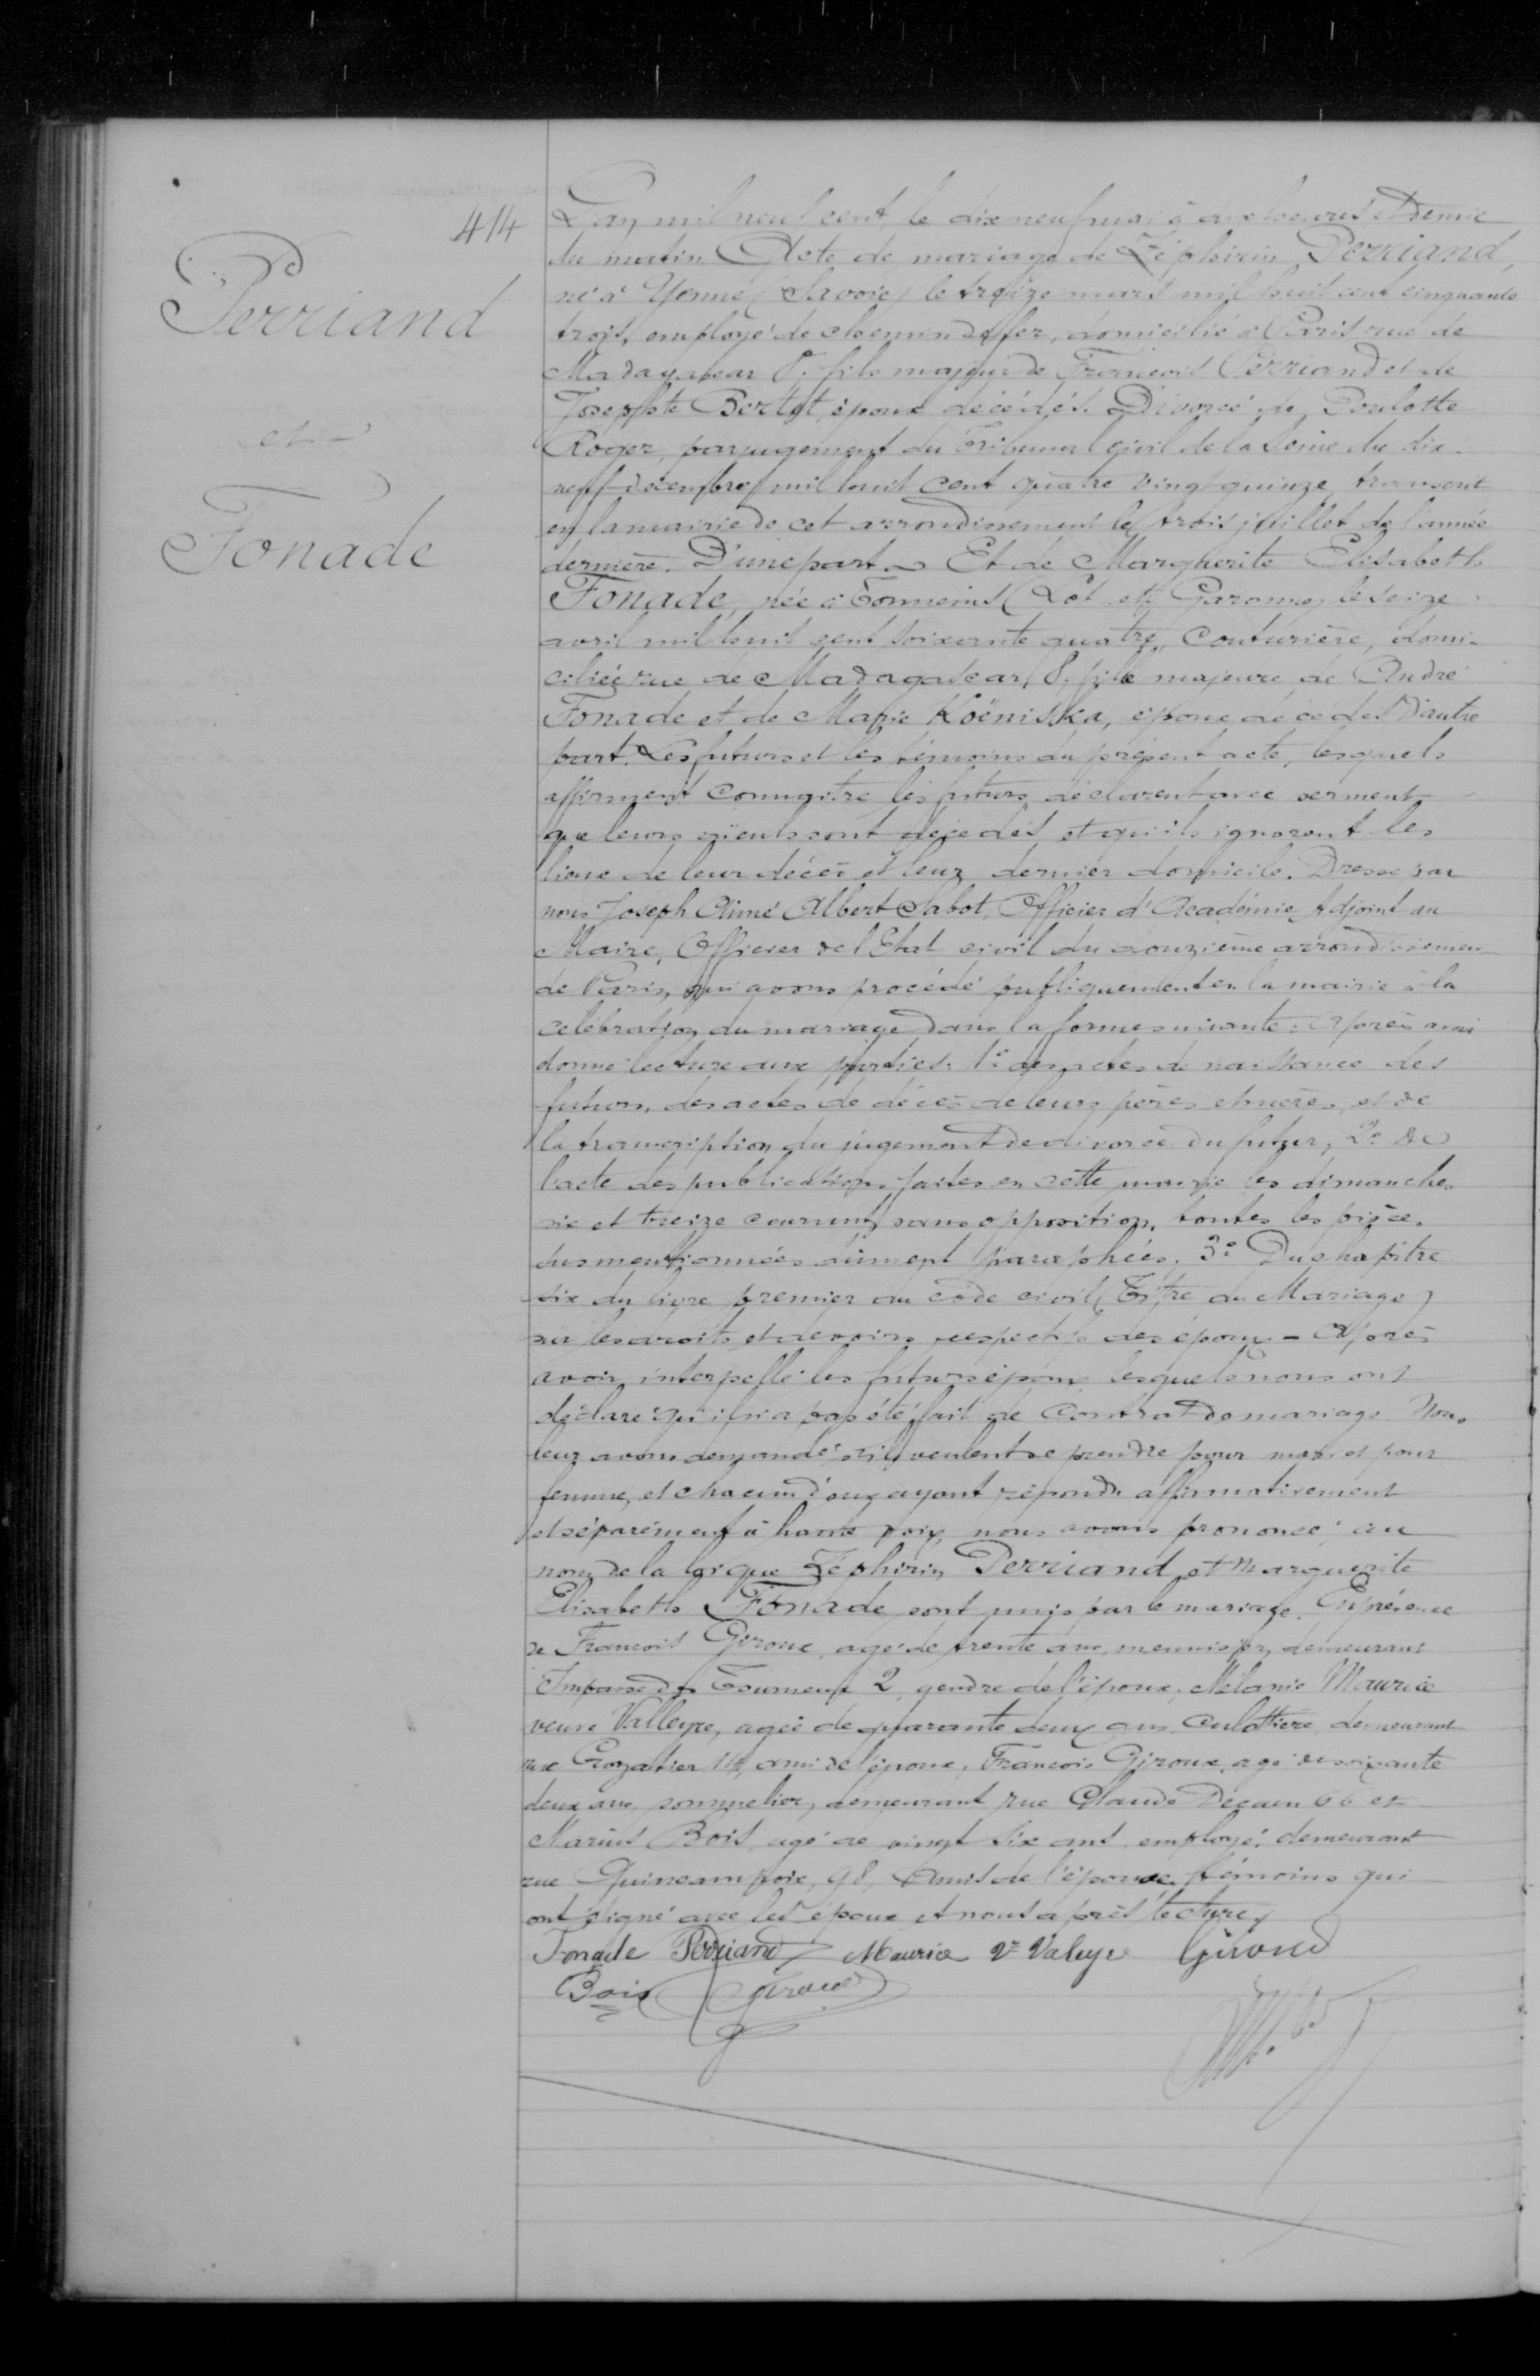414
Perriand
et
Fonade
L'an mil neuf cent le dix neuf mai à dix heures et Demie
du matin Acte de mariage de Zéphirin Perriand,
né à Yonne (Saone) le treize mars mil huit cent cinquante
trois, employé de chemin de fer, domicilié à Paris rue de
Madagascar fils majeur de François Perriand et de
Josephte Bertot, époux décédés. Divorcé de Porlotte
Roger, par jugement du Tribunal civil de la Seine du dix
neuf decembre mil huit cent quatre vingt quinze transcrit
en la mairie de cet arrondissement le trois juillet de l'année
dernière. D'une part. Et de Marguerite Elisabeth
Fonade, née à Tonnems (Lôt et Garonne) le seize
avril mil huit cent soixant quatre couturière domi-
ciliée rue de Madagascar 8, fille majeure de André
Fonade et de Marie Koeniska, epoux décédé et d'autre
part Les futurs et les témoins du présent acte, lesquels
affirmant connaitre les futurs déclarent avec serments
que leurs aïeuls sont décédé et qu'ils ignorent les
lieux de leur décès et leur dernier domicilie. Dressé par
nous Joseph Aimé Albert Labot, Officier d'Académie Adjoint au
Maire, Officier de l'Etat-Civil du douzième arrondissement
de Paris, qui avons procédé publiquement en la mairie à la
célébration du mariage dans la forme suivante: Après avoir
donne lecture aux parties 1° des actes de naissance des
fonctions, des actes de décès de leurs pères et mères et de
la transcription du jugement de divorce du futur 2° de
l'acte des publications faites en cette marie lies dimanche
six et treize courant sans opposition, toutes les pièce
sus mentionnées dûment paraphées 3° Du chapitre
six du livre premier du Code Civil (Titre du Mariage)
sur les droits et devoirs; respectifs des époux-Après
avoir interpellé les futurs époux lesquels nous ont
déclaré qu'il n'a pas été fait de contrat de mariage. Nous
leur avons demande s'ils veulent se prendre pur mari et pour
femme, et chacun d'eux ayant répondu affirmativement
et séparèment à haute voix, nous avons prononcé au
nom de la loi que Zéphirin Perriand et Marguerite
Elisabetta Fonade sont unis par le mariage. En présence
de Francois Giroux, agè de trente ans, menuisier, demeurant
Impasse de Tameux 2, gendre del'époux; Mélanie Maurice
veuve Valleyre, agèe de quarante deux ans, Cullotière, demeurant
rue Gozalier 14 ami de l'époux, Francois Giroux, agè de soixante
deux ans, sommelier, demeurant rue Claude Decain 66 et
Marius Bois, agé de vingt six ans, employé demeurant
rue Quincampoix, 98, Amis de l'épouse, témoins qui
ont signé avec les époux et nous après lecture.
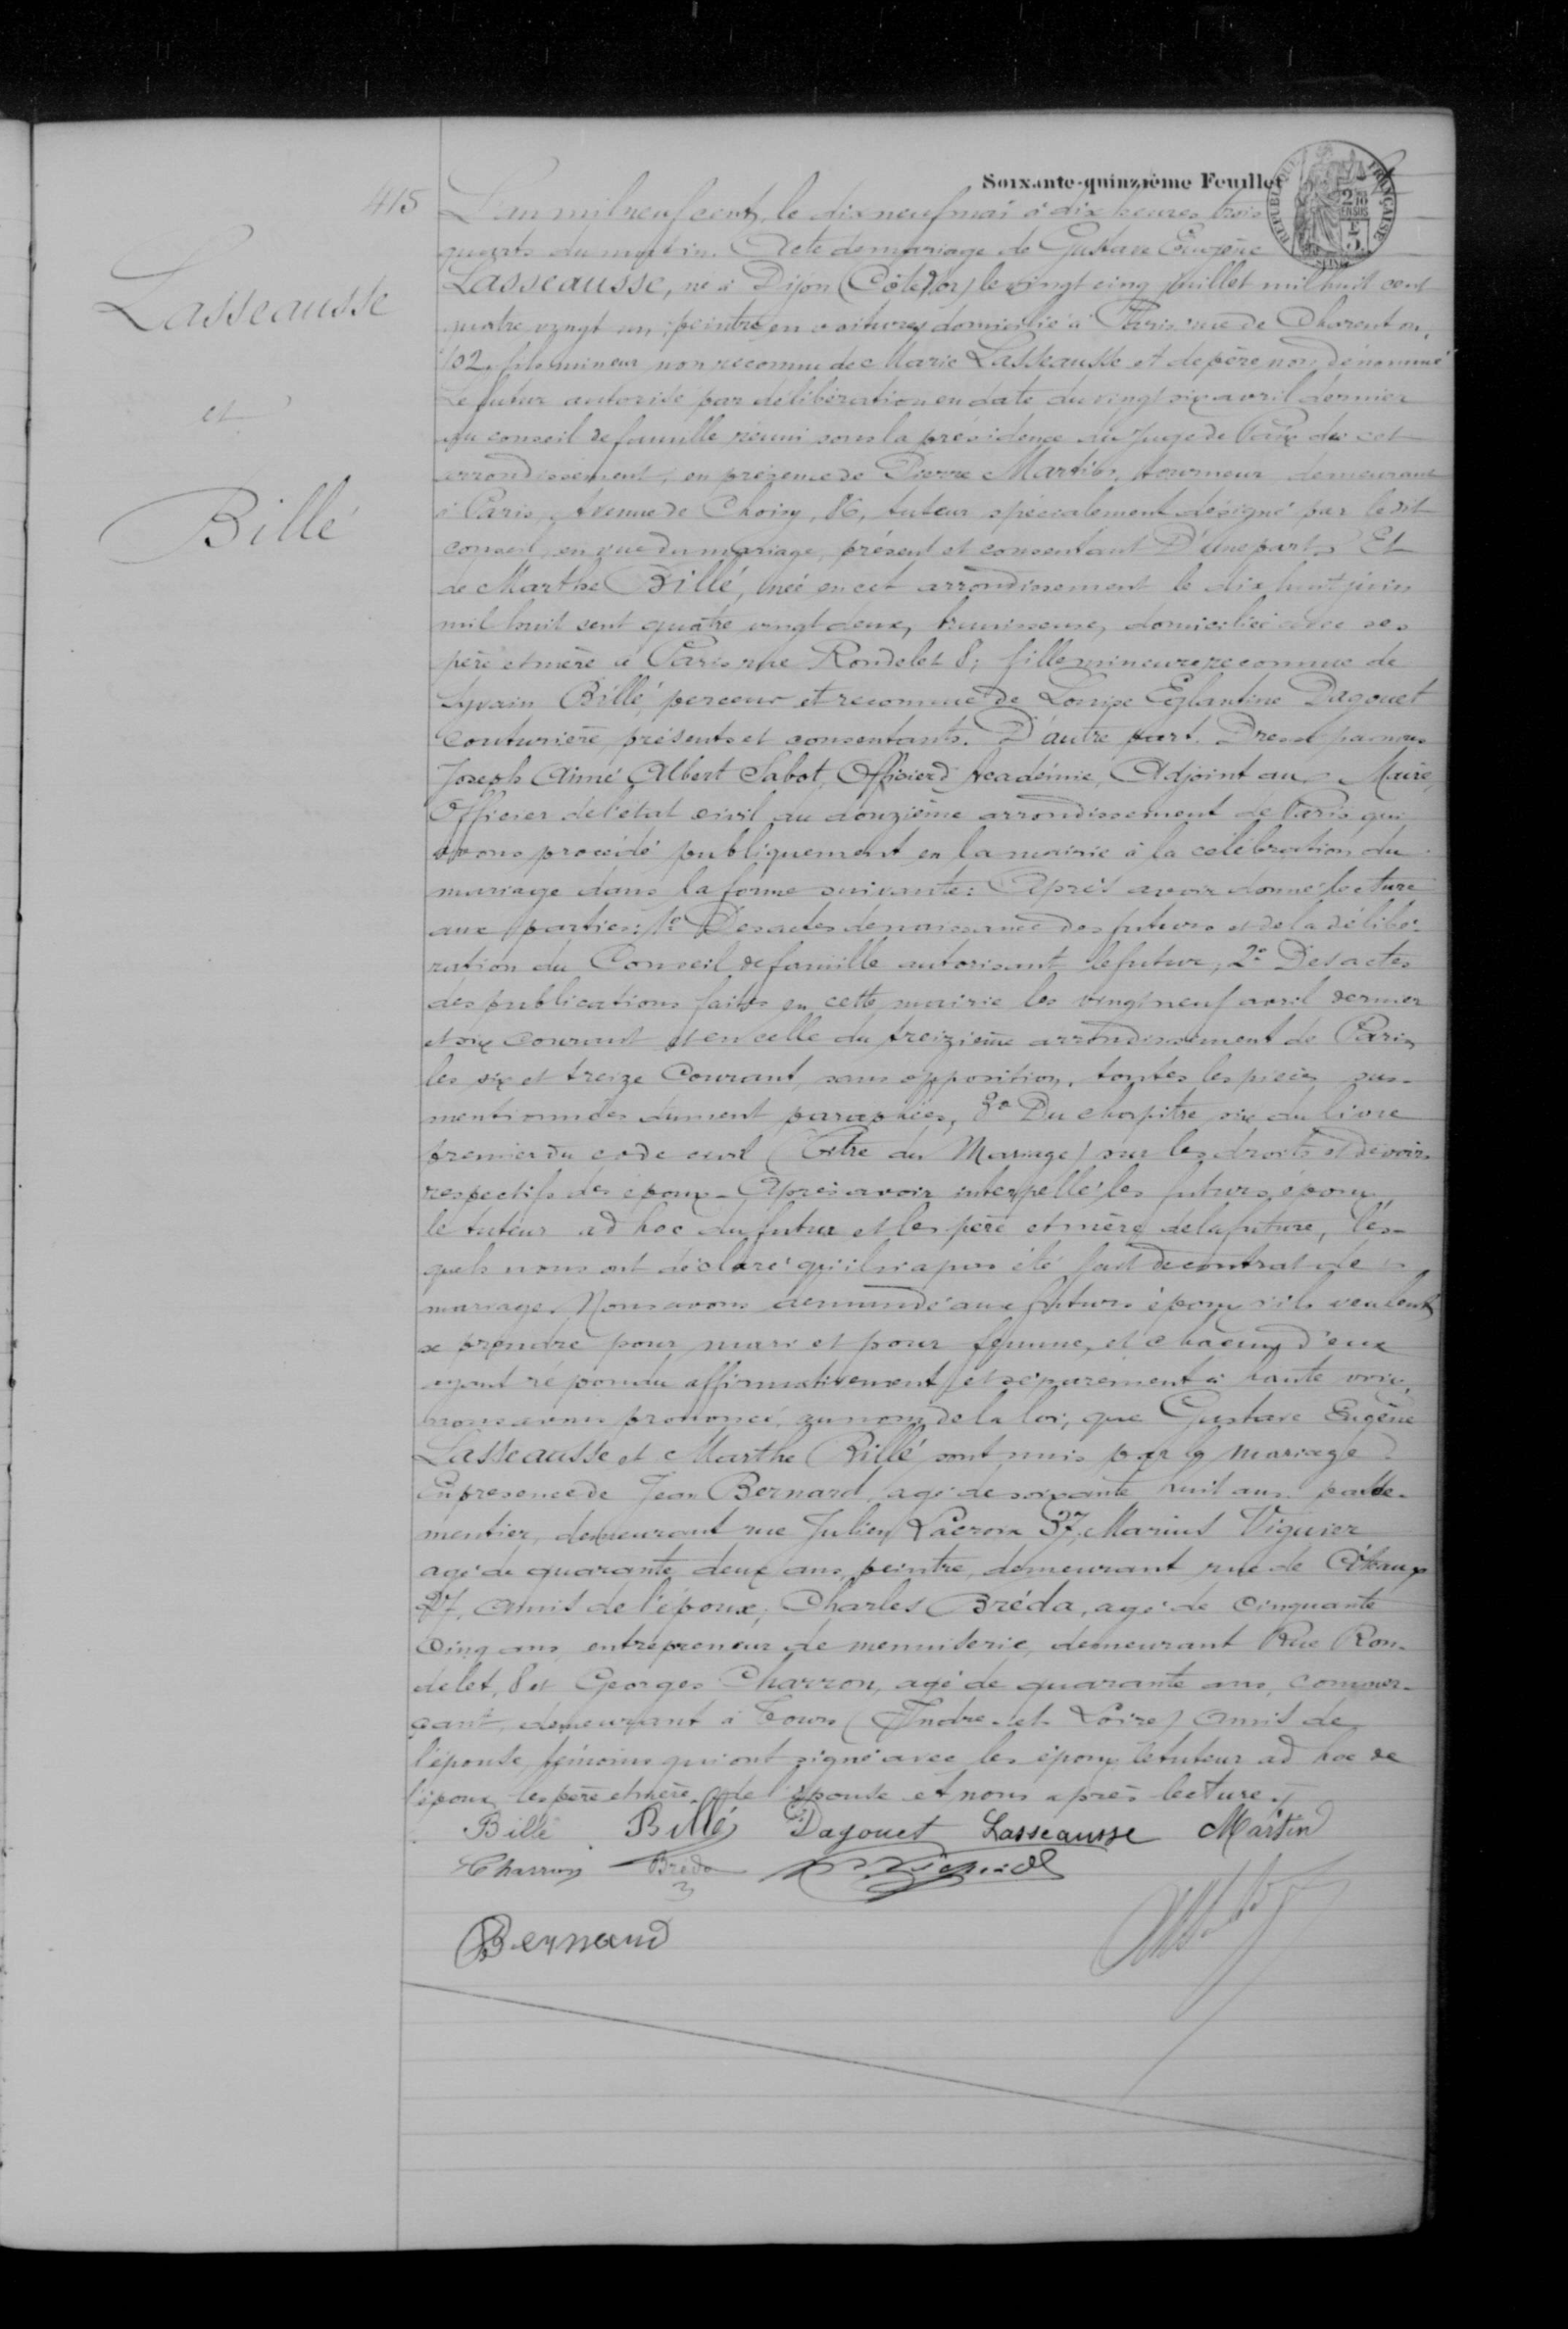415
Lasseausse
et
Bille
L'an mil neuf cent, le dix neuf mai a dix heures trois
quarts du matin. Acte de mariage de Gustave Eugène
Lasseausse, né à Dijon (Côte d'or) le vingt cinq juillet mil huit cent
quatre vingt an peintre en voitures, domicilié à Paris rue de Charenton
102, fils mineur, non reconnu de Marie Lasseauble et de père non dénommé
Le futur autorisé par délibération en date du vingt six avril dernier
du conseil de famille renvoi sous la présidence du Juge du Paix de cet
arrondissement en présence de Pierre Martier, tourneur demeurant
à Paris, Avenue de Coisy 86, tuteur spécialiement désigne par le dit
conseil en une du mariage, présent et consentant D'une part. Et
de Martine Billé, née en cet arrondissement, le dix huit juin
mil huit cent quatre vingt deux, brunisseuse, domiciliée chez ses
père et mère à Paris rue Rondelet 8; fille mineure reconnue de
Sylvain Brillé, perceur et reconnue de Louise Eglantine Dagouet
couturière, présents et consentants. D'autre part. Dressé par nous
Joseph Aimé Albert Sabot Officier d'académie, Adjoint au Maire,
Officier del'etat civil du douzième arrondissement de Paris qui
avons procédé publiquement en la mairie à la célébration du
mariage dans la forme suivante: Après avoir donné lecture
aux parties: 1° Des actes de naissance des futurs et de la délibé-
ration du Conseil de famille autorisant le futur, 2-Des actes
des publications faites en cette mairie le vingt neuf avril dernier
etsix courant et en celle du treizieme arrondissement de Paris
les dix et treize courant, sans opposition, toutes les pièces sus-
mentionnées dûment paraphées, 3° Du chapitre six du livre
premier du code civil (Titre du Mariage) sur les droits et devoirs
respectifs des époux Après avoir interpellé les futurs époux
le tuteur ad hoc du futur et les père et mère de la futurs, les-
quels nous ont déclaré qu'il n'a pas été fait de contrat de
mariage. Nous avons demandé aux futurs époux s'ils veulent
se prendre pour marie et pour femme, et chacun d'eux
ayant répondu affirmativement et séparément à haute voix
nous avons prononcé au nom de la loi, que Gustave Eugène
Lasseausse et Marth Rillé sont unis par le mariage.
En présence de Jean Bernard agé de soixante huit ans, passe
mentier, demeurant rue Julien Lacroix 37, Marius Vignier
agé de quarante deux ans, peintre demeurant rue de Cétaux
27, Amis de l'époux, Charles Bréda, agé de cinquante
cinq ans, entrepreneur de menuiserie, demeurant Rue Ron-
delet, 8 et Georges Charron, agé de quarante ans, commer-
çant demeurant à Tours (Indre-et-Loire) Amis de
l'épouse, témoins qui ont signé avec les époux le tuteur ad hoc de
l'époux, le père et mère de l'épouse et nous après lecture.